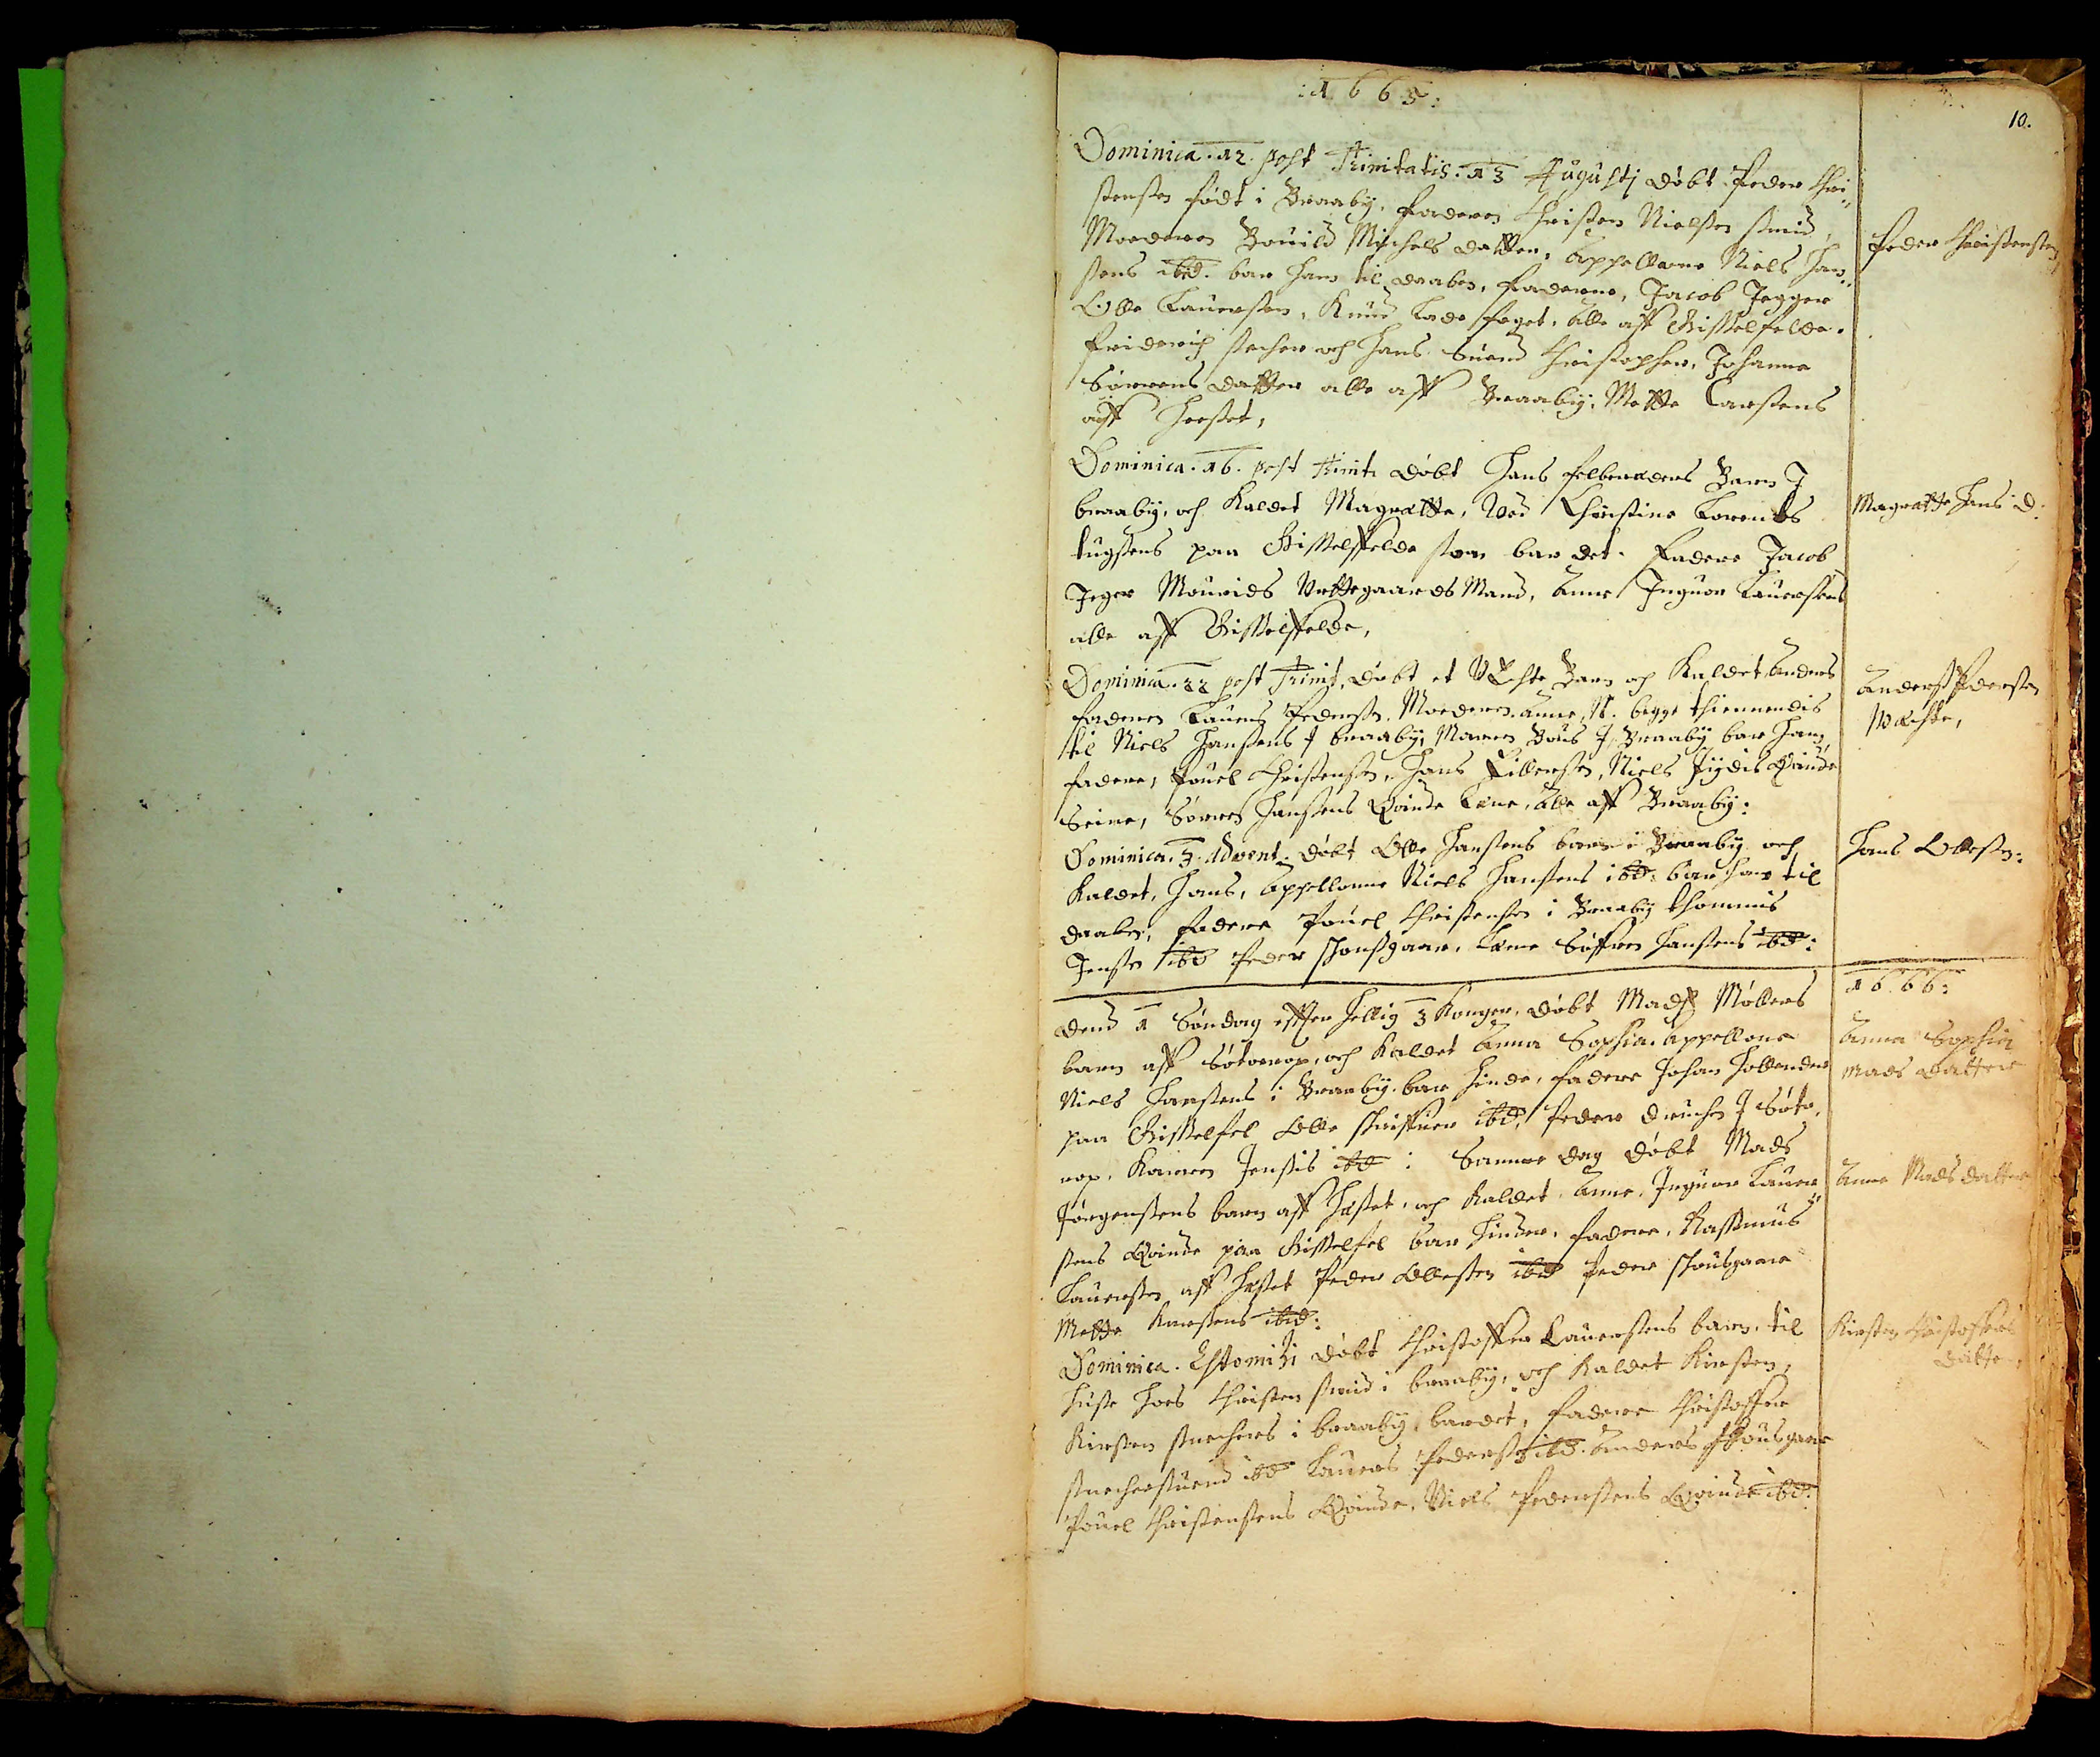1665:
10.
Dominica 12 Post Trinitatis 13. Augustj døbt Peder Chri¬
stensen født i Braaby. Faderen Christen Nielsen Smid,
Moederen Bouild Michals datter, Appellone Niels Jens¬
ens ibid. bar Ham til daaben. Faderne, Jacob Jegger
Olle Lauersen, Knud Ladefoget, Alle aff Gisselfelde.
Friderich Jensen och Hans Suend Christopher. Johanne
Sørrens Datter alle aff Braaby. Mette Carstens
aff Herset.
Peder Christensen
Dominica 16. Post Triniti. døbt Hans Felberæders Barn J
Braaby, och kaldet Magrætte. Mod. Christine Laurids
tugsters## paa Gisselfelds som bar det. Fadere Jacob
Jeger Mourids Vættegaards## Mand, Anne Jnguer Lauersten
alle aff Gisselsfelde,
Magrethe Hansd:
Dominica. 22 Post Trinit. døbt et UEchte Barn och kaldet Anders
Faderen Lauris Pedersen. Moederen Anne N. begge thiennendis
til Niels Hansens J Braaby. Maren Bous J Braaby bar Ham
Fadere, Pouel Christensen, Hans Eillersen. ## Niels Jydis Qvinde
Seine, Søren Hanssøns Qvinde Læne alle aff Braaby:
Anders Pedersen 
Uæchte
Dominica. 3. Advent. døbt Olle Hanssens barn i Braaby och
kaldet Hans, Appellones Niels Hanssens i ##: bar ham til
Daaben; Fadere Pouel Christensen i Braaby. Thommis
Jensen ibd Peder Stensgaar Læne Søfren Hanssens ibd:
Hans Ollessen##
Dend 1 Søndag effter Hellig 3 Konger, døbt Mads Møllers
barn aff Søtorup, och kaldet Anne Sophie. Appellone
Niels Hansens i Braaby. bar hinde, Fadere Johan Hollender
paa Gisselfel Olle Christen ibd. Peder Druches, J Søto¬
rup. Karren Jensis ibd:
1666:
Anne Sophie 
Madsdatter
Samme dag døbt Mads 
Jørgensøns barn aff Hæset och kaldet Anne. Ingmer## Laur¬
sens Qvinde paa Gisselfel bar Hinder. Fadere, Rassmus
Lauersen aff Hæset. Peder Ollissen ibd. Peder Stousgaars,
Mette Karstens ibd:
Anne Madsdatter
Dominica. Estomihi. døbt Christoffer Lauersens barn til
huse hoes Christen Smid i Braaby och kaldet Kirsten,
Kirsten Snechers i Braaby bar det. Fadere Christoffer
Snechersuend ibd. Lauers Pedersen ibd. Anders Skousgaar
Pouel Christensens Qvinde, Niels Pedersens Qvinde ibd:
Kirsten Christoffers 
datter